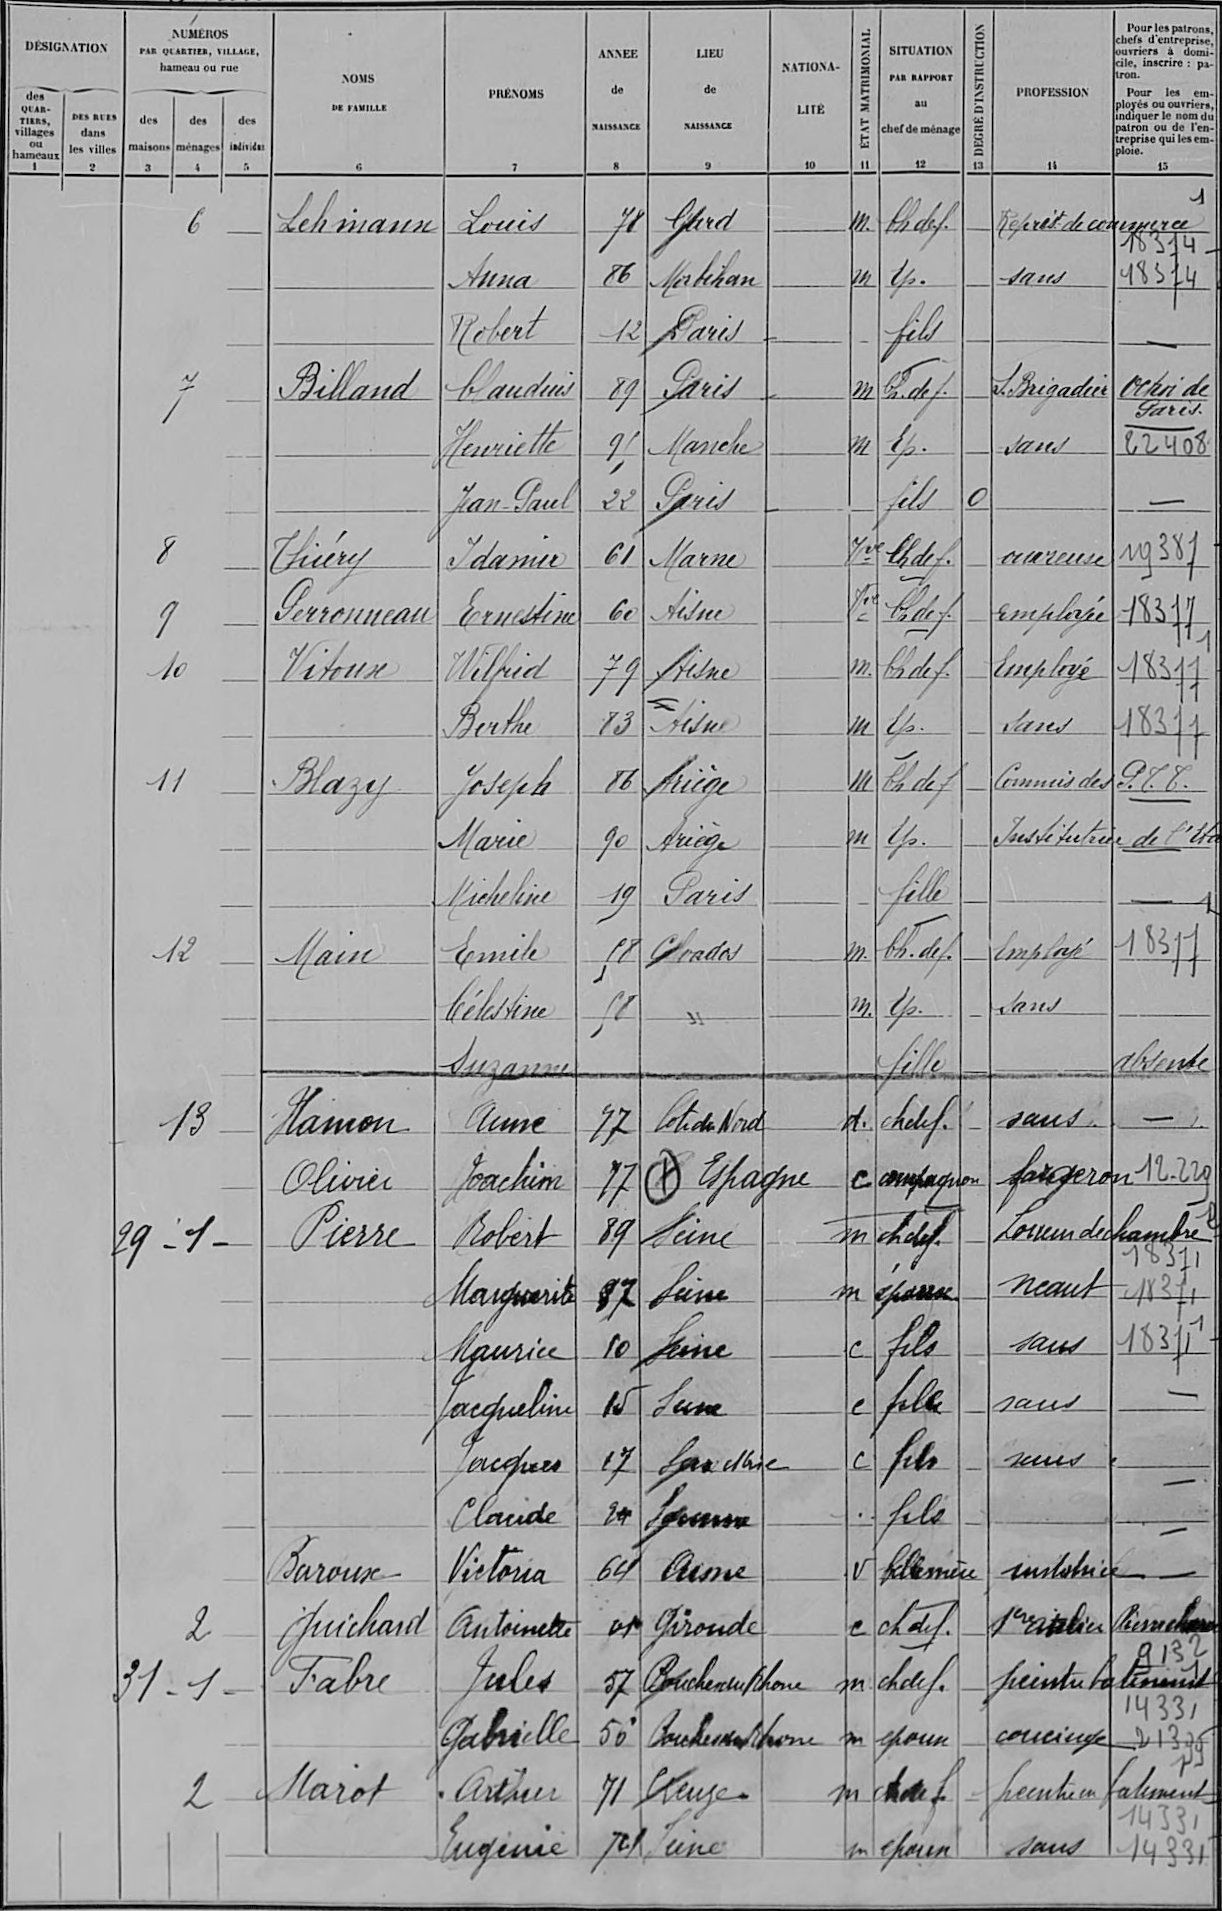Lehmann/Louis/78/Gard/¤/m /Ch de f /¤/Représt de commerce/?183741
¤/Anna/86/Morbihan/¤/m/Ep /¤/sans/18374
¤/Robert/12/Paris/¤/¤/fils/¤/¤/¤
Billaud/Claudius/89/Paris/¤/m/Ch de f /¤/S Brigadier/octroi de Paris
¤/Henriette/95/Manche/¤/m/Ep /¤/sans/22408
¤/Jean-Paul/22/Paris/¤/¤/fils/O/¤/¤
Thiéry/Idamir/61/Marne/¤/Vve/Ch de f /¤/ouvreuse/19387
Perronneau/Ernestine/60/Aisne/¤/Vve/Ch de f /¤/employée/183771
Vitoux/Wilfrid/79/Aisne/¤/m /Ch de f /¤/employé/18377
¤/Berthe/83/Aisne/¤/m/Ep /¤/sans/18377
Blazy/Joseph/86/Ariège/¤/m/ch de f/¤/commis des /P T T
¤/Marie/90/Ariège/¤/m/Ep /¤/Institutrice /de l'éta
¤/Micheline/19/Paris/¤/¤/fille/¤/¤/¤
Main/Emile/58/Calvados/¤/m /ch de f /¤/employé/18377
¤/Célestine/58/¤/¤/m /Ep /¤/sans/¤
¤/Suzanne/¤/¤/¤/¤/fille/¤/¤/absente
Hamon/Aime/97/Cote du Nord/¤/d /ch de f /¤/sans/¤
Olivier/Joachim/97/Espagne/¤/c/compagnon/¤/forgeron/12 229
Pierre/Robert/89/Seine/¤/m/ch def /¤/Loueur de chambre/?18371
¤/Marguerite/87/Seine/¤/m/épouse/¤/neant/18371
¤/Maurice/10/Seine/¤/c/fils/¤/sans/183711
¤/Jacqueline/15/Seine/¤/c/fille/¤/sans/¤
¤/Jacques/17/Sarthe/¤/C/fils/¤/sans/¤
¤/Claude/24/Lorraine/¤/¤/fils/¤/¤/¤
Baroux/Victoria/64/Aisne/¤/V/belle mère/¤/institutrice/¤
Guichard/Antoinette/01/Gironde/¤/c/ch def /¤/1ere atelier/Pierre ?9132
Fabre/Jules/57/Bouches du Rhone/¤/m/ch de f /¤/peintre batiment/?14331
¤/Gabrielle/50/Bouches du Rhone/¤/m/épouse/¤/concierge/21399
Marot/Arthur/71/Creuse/¤/m/ch de f/¤/peintre en batiment/?14331
¤/Eugénie/74/Seine/¤/m/épouse/¤/sans/14331
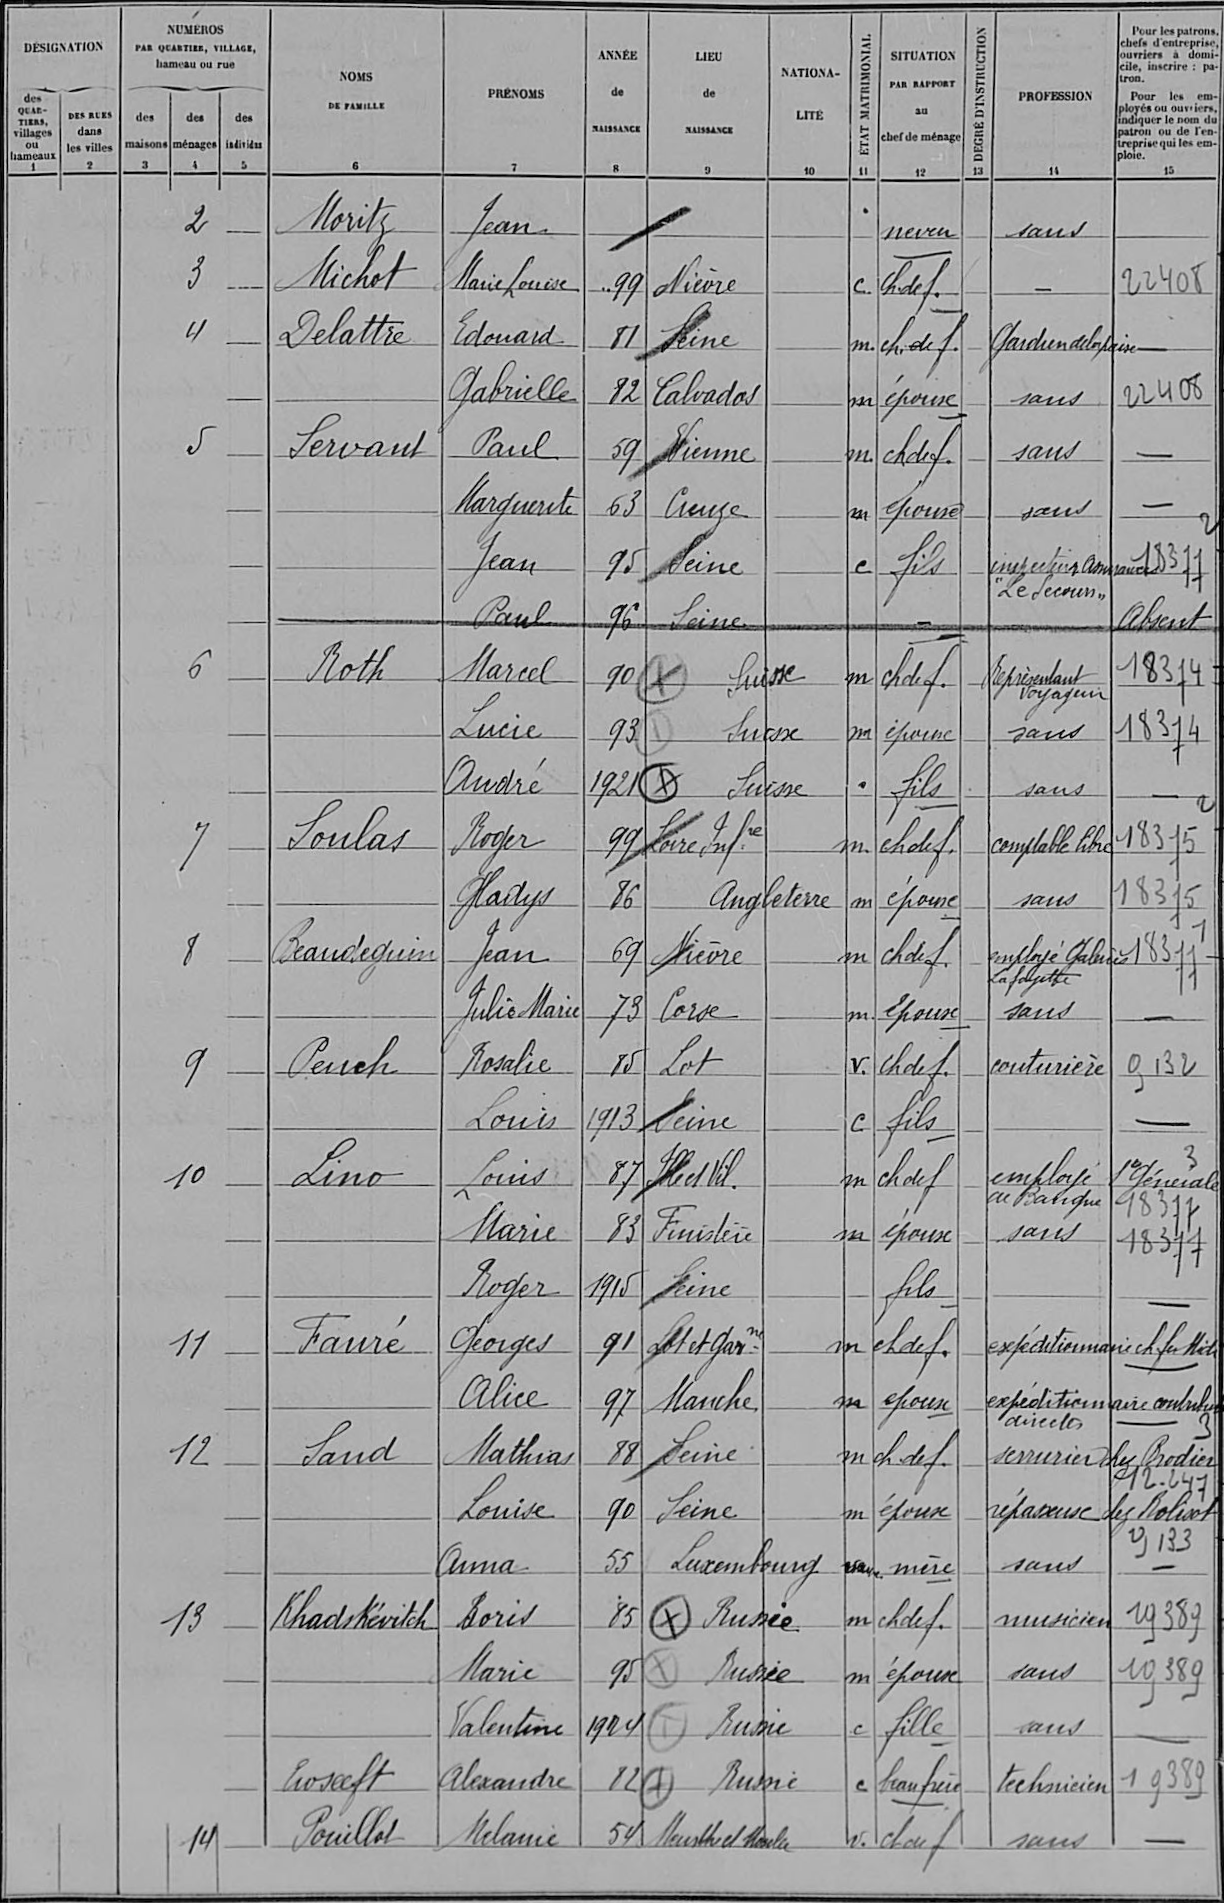Moritz/Jean/¤/¤/¤/¤/neveu/¤/sans/¤
Michot/Marie Louise/99/Nièvre/¤/C /ch de f /¤/¤/22408
Delattre/Edouard/81/Seine/¤/m /ch de f /¤/Gardien de la paix/¤
¤/Gabrielle/82/Calvados/¤/m/épouse/¤/sans/22408
Servant/Paul/59/Vienne/¤/m /ch de f /¤/sans/¤
¤/marguerite/63/Creuse/¤/m/épouse/¤/sans/¤
¤/Jean/95/Seine/¤/C/Fils/¤/inspecteur Assurances !"Le secours"/18377
¤/Paul/96/Seine/¤/¤/¤/¤/¤/absent
Roth/Marcel/90/Suisse/¤/m/ch de f /¤/représentant!voyageur/18374
¤/Lucie/93/Suisse/¤/m/épouse/¤/sans/18374
¤/André/1921/Suisse/¤/¤/fils/¤/sans/¤
Loulas/Roger/99/Loire Infre/¤/m/ch de f /¤/comptable libre/18375
¤/Gladys/86/Angleterre/¤/m/épouse/¤/sans/18375
Beaudeguin/Jean/69/Nièvre/¤/m/ch de f /¤/employé galeries!Lafayette/18377
¤/Julie Marie/73/Corse/¤/m/épouse/¤/sans/¤
Peuch/Rosalie/85/Lot/¤/V /ch de f /¤/couturière/9132
¤/Louis/1913/Seine/¤/C/fils/¤/¤/¤
Lino/Louis/87/Ile et Vil /¤/m/ch de f/¤/employé!de banque/Se générale!18377
¤/Marie/83/Finistère/¤/m/épouse/¤/sans/18377
¤/Roger/1915/Seine/¤/¤/fils/¤/¤/¤
Fauré/Georges/91/Lot et garne/¤/m/ch de f /¤/expéditionnaire ch fer Midi/¤
¤/Alice/97/Manche/¤/m/épouse/¤/expéditionnaire contribut !directes/¤
Sand/Mathias/88/Seine/¤/m/ch de f /¤/serrurier chez brodier/!12 247
¤/Louise/90/Seine/¤/m/épouse/¤/repasseuse chez Rolisot/!9133
¤/Anna/55/Luxembourg/¤/veuve/mère/¤/sans/¤
Khadshévitch/Boris/85/Russie/¤/m/ch de f /¤/musicien/19389
¤/Marie/95/Russie/¤/m/épouse/¤/sans/19389
¤/Valentine/1924/Russie/¤/c/fille/¤/sans/¤
Proseeft/Alexandre/82/Russie/¤/c/beau frère/¤/technicien/19389
Pouillot/Melanie/54/Meurthe et Moselle/¤/v /ch du f/¤/sans/¤